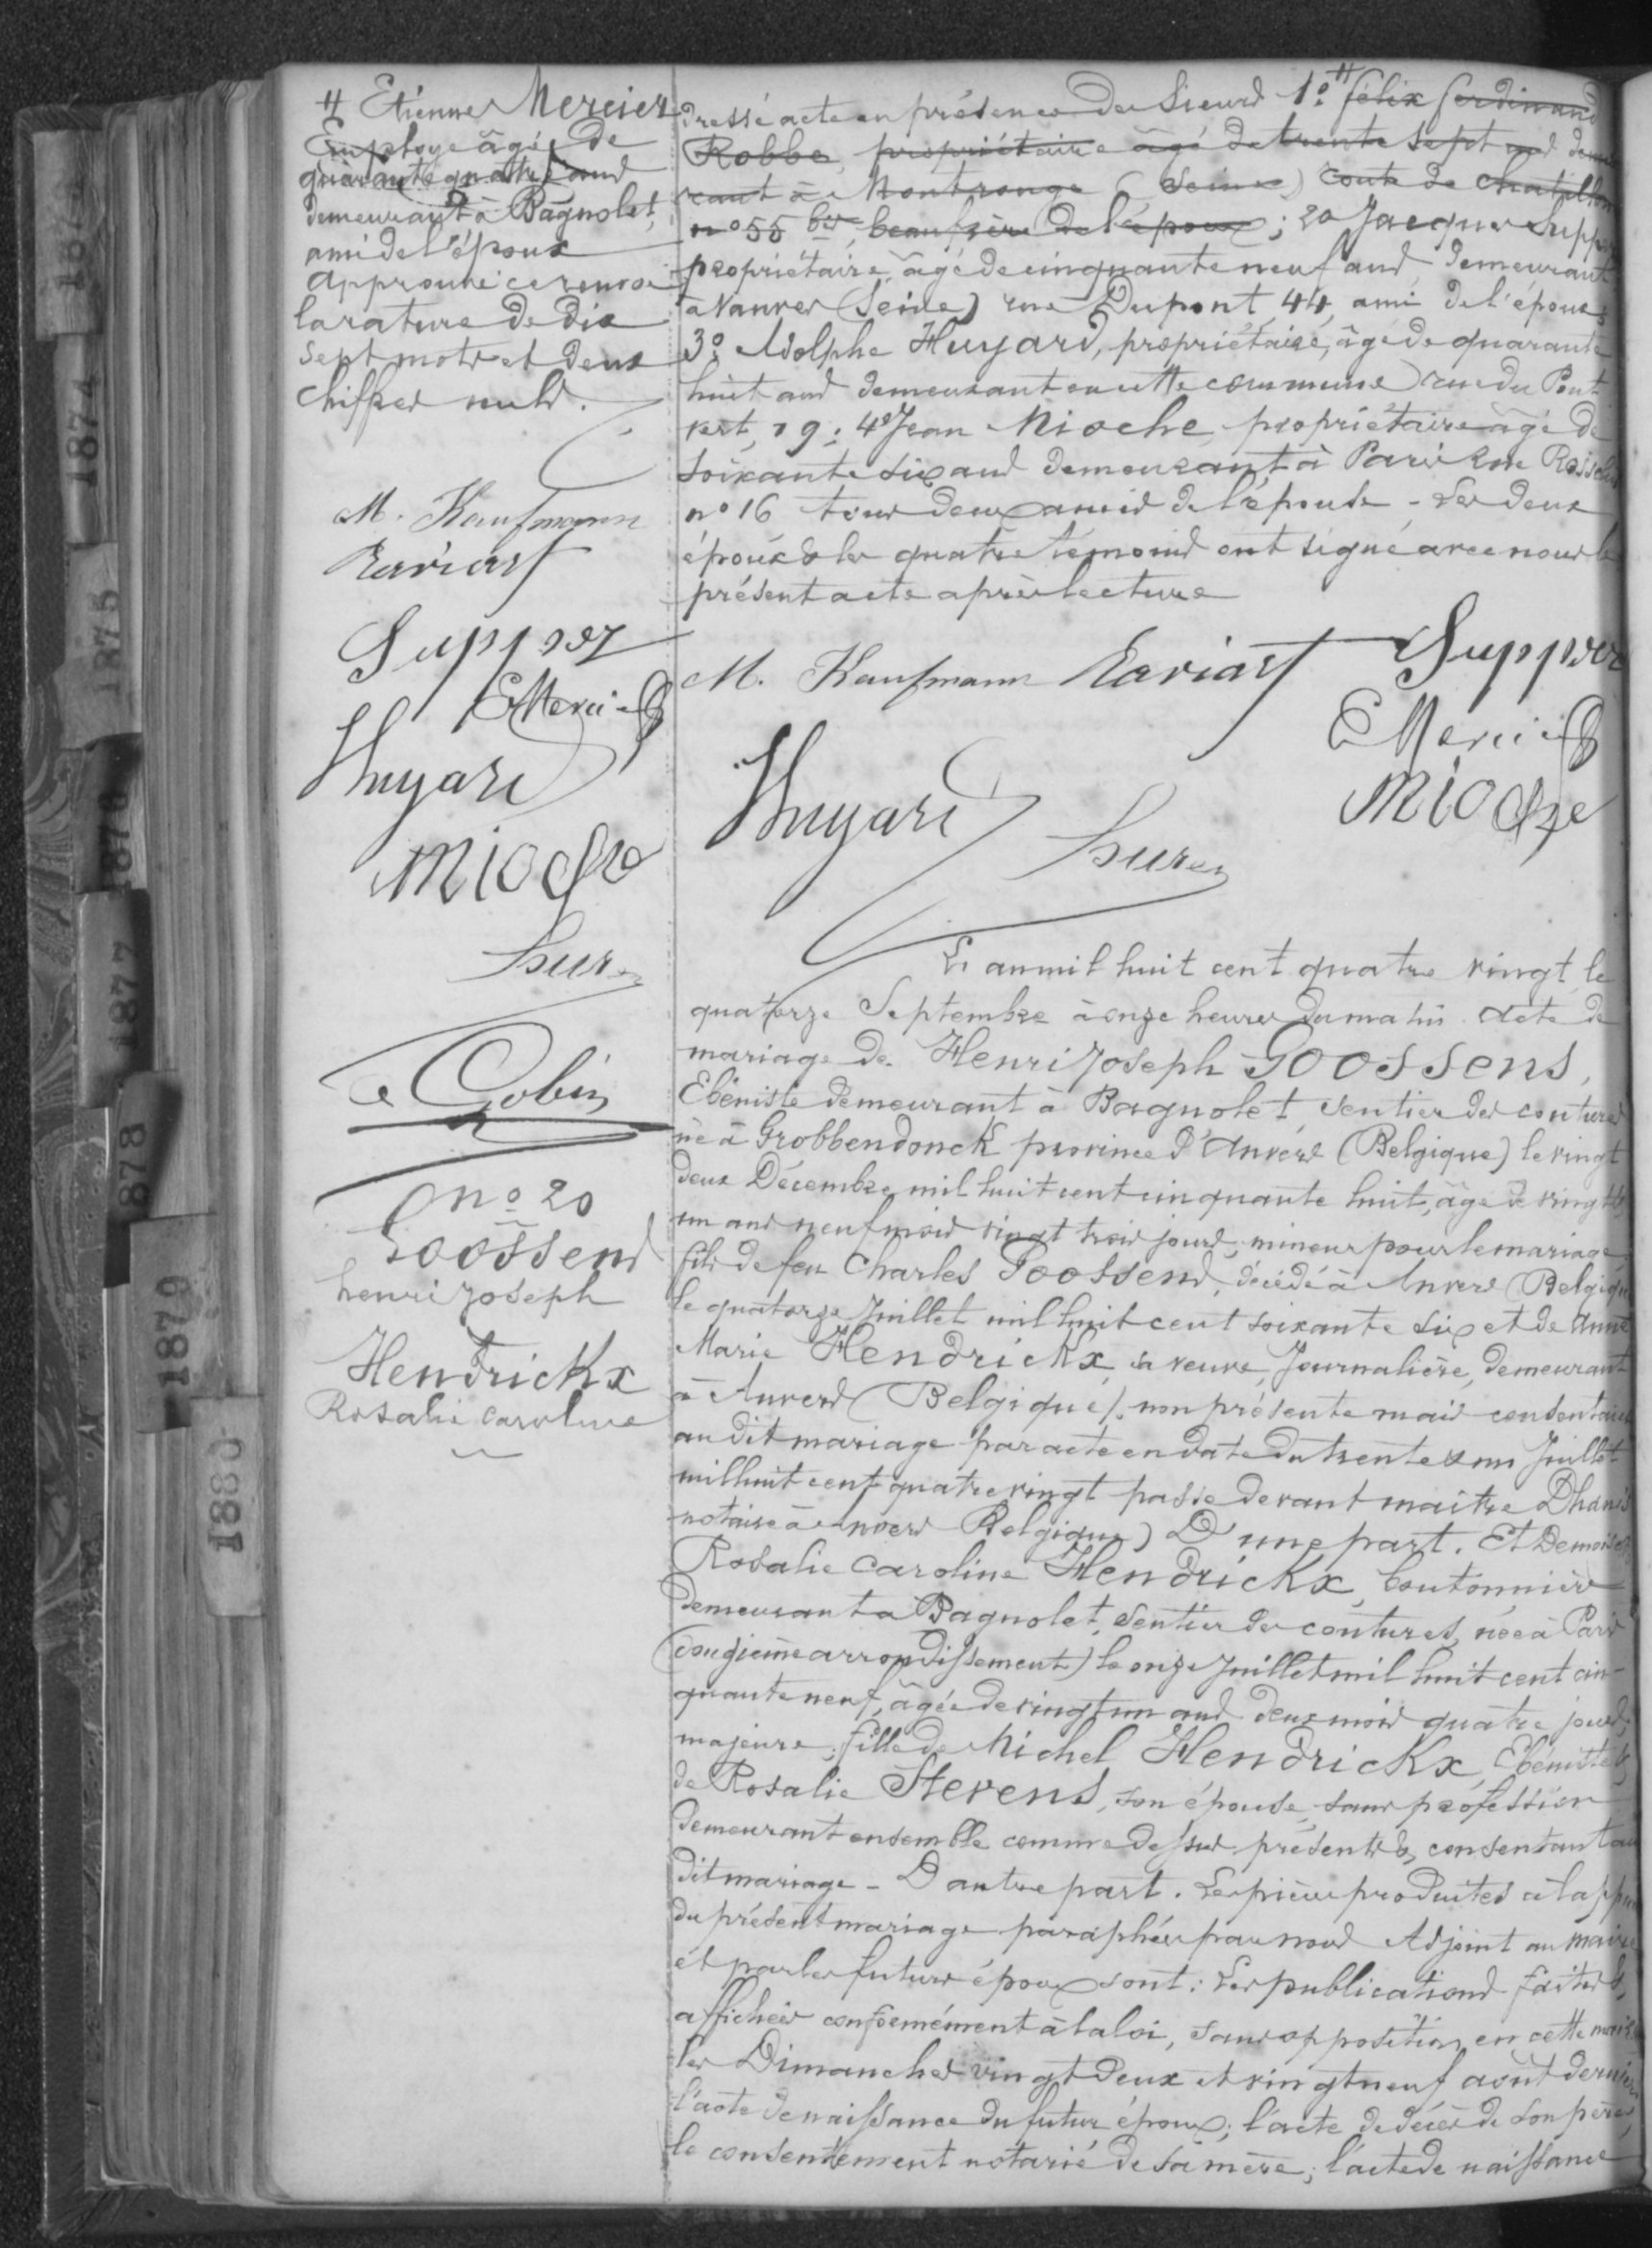Dressé acte en présence du sieur 1: felix ferdinand
Robbe propriétaire âgé de trente sept ans demeu
rant à Montrouge (Seine) route de Chatillon
n°55 bis beau frère de l'époux; 2° Jacques Suppe
propriétaire âgé de cinquante neuf ans demeurant
à Vannes (Seine) rue Dupont 44, ami de l'épouse
3° Adolphe Huyard, propriétaire âgé de quarante
huit ans demeurant en cette commune rue du Pont
vert, 19; 4° Jean Nioche propriétaire âgé de
soixante dix ans demeurant à Paris Rue Rassek
N°16, tout deux amis de l'épouse-les deux
époux et les quatre témoins ont signé avec nous le
présent acte après lecture
N°20
Poossens
henri joseph
Hendrick x
Rosalie caroline
L'an mil huit cent quatre vingt le
quatorze Septembre à onze heures du matin date du
mariage de Henri Joseph Poossens,
Ebéniste demeurant à Bagnolet, sentier des coutures
né à Probbendonck province d'Anvère (Belgique) le vingt
deux Décembre mil huit cent cinquante huit, âgé de vingt et
un ans neuf mois vingt trois jours, mineur pour le mariage
fils de feu Charles Poosens, décédé à Anvers (Belgique)
le quatorze juillet mil huit cent soixante six et de Anne
Marie Hendrick sa veuve, Journalière, demeurant
à Anvers (Belgique) non présente mais consentante
audit mariage par acte en date du trente un Juillet
mil huit cent quatre vingt passé devant maître Dhanis
notaire à Anvers (Belgique) D'une part. Et Demoiselle
Rosalie Caroline Hendrickx, Cantonnière
demeurant à Bagnolet sentier des coutures, née à Paris
(douzième arrondissement) le onze Juillet mil huit cent cin-
quante neuf, âgée de vingt un ans deux mois quatre jours,
majeure; fille de Michel Hendrickx, Ebéniste et
de Rosalie Hevens, son épouse, sans profession
demeurant ensemble comme de sus présente & consentant au
dit mariage-D'autre part. Les pièces produites à l'appui
du présent mariage paraphées par nous Adjoint au maire
et par les futurs époux sont: Les publications faites et
affichées conformément à la loi, sans opposition en cette mairie
les Dimanches vingt deux et vingt neuf août dernier
l'acte de naissance du futur époux; l'acte de décès de son père
le consentement notarié de sa mère, l'acte de naissance
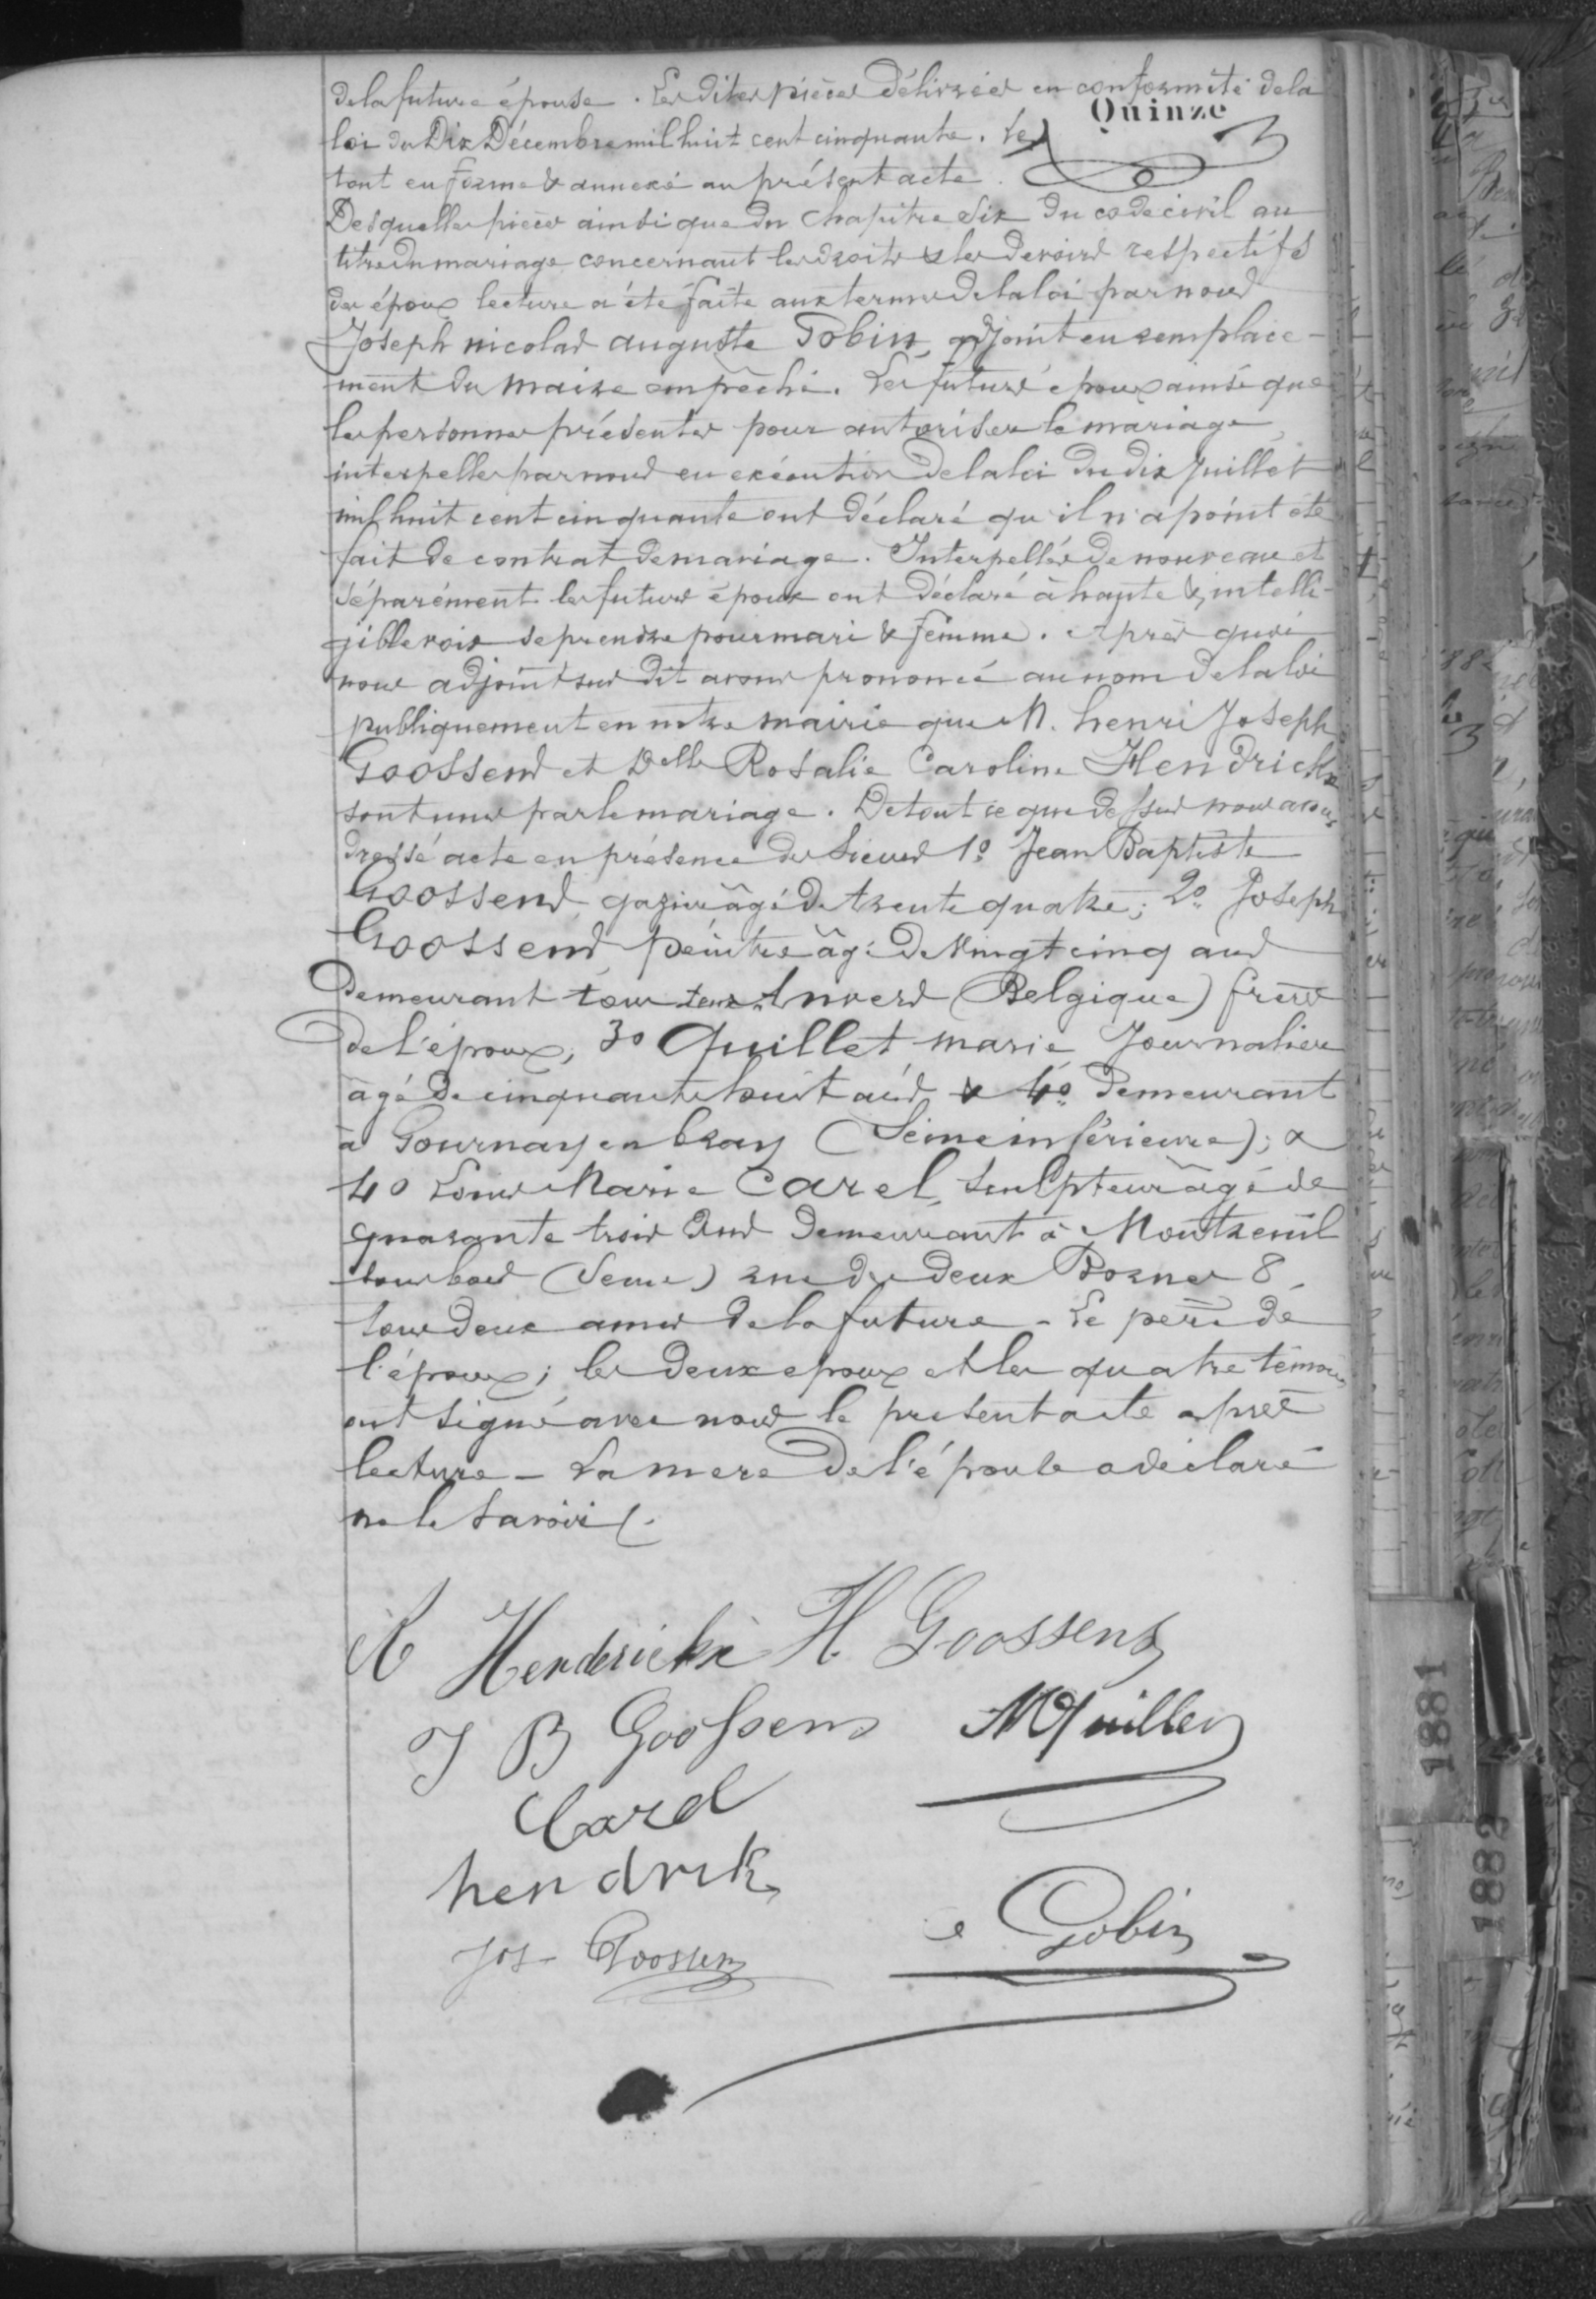de la future épouse. Lesdites pièces délivrées en conformité dela
loi du Dix Décembre mil huit cent cinquante. Le
tout en forme & annexé au présent acte.
Desquelles pièces ainsi que du chapitre six du code civil au
titre du mariage concernant les droits & les devoirs respectifs
des époux lecture a té fait aux termes de la loi par nous
Joseph nicolas auguste Gobin, adjoint en remplace-
ment du maire empêché. Les futurs époux que
les personnes présentes pour autoriser le mariage
interpeller par nous en création de la loi du dix juillet
mil huit cent cinquante ont déclaré qu'il n'a point été
fait de contrat de mariage. Interpellé de nouveau et
séparément les futurs époux ont déclaré à haute & intelli-
gible voix se prendre pour mari & femme. Après quoi
nous adjoint susdit avons prononcé au nom de la loi
publiquement en notre mairie que M. henri Joseph
Poossens et Delle Rosalie Caroline Hendrick
sont unis par le mariage. De tout ce que dessus nous avons
dressé acte en présence du sieur 1°: Jean Baptiste
Poossens gaziere âgé de trente quatre; 2°: Joseph
Poossens, peintre âgé de vingt cinq ans
demeurant tous deux à Anvers (Belgique) frère
de l'époux, 3° Quillet Marie Journaliere
âgé de cinquante huit ans 4° demeurant
à Gournay en Bray (Seine inférieure); &
4° Louis Marie Carel, sculpteur âgé de
quarante trois ans demeurant à Montreuil
sous boi (Seine) rue du deux Pornes 8,
tous deux amis de la future. Le père de
l'époux les deux epoux et les quatre témoins,
ont signé avec nous le présent acte après
lecture. La mère de l'épouse a déclaré
ne le savoir.